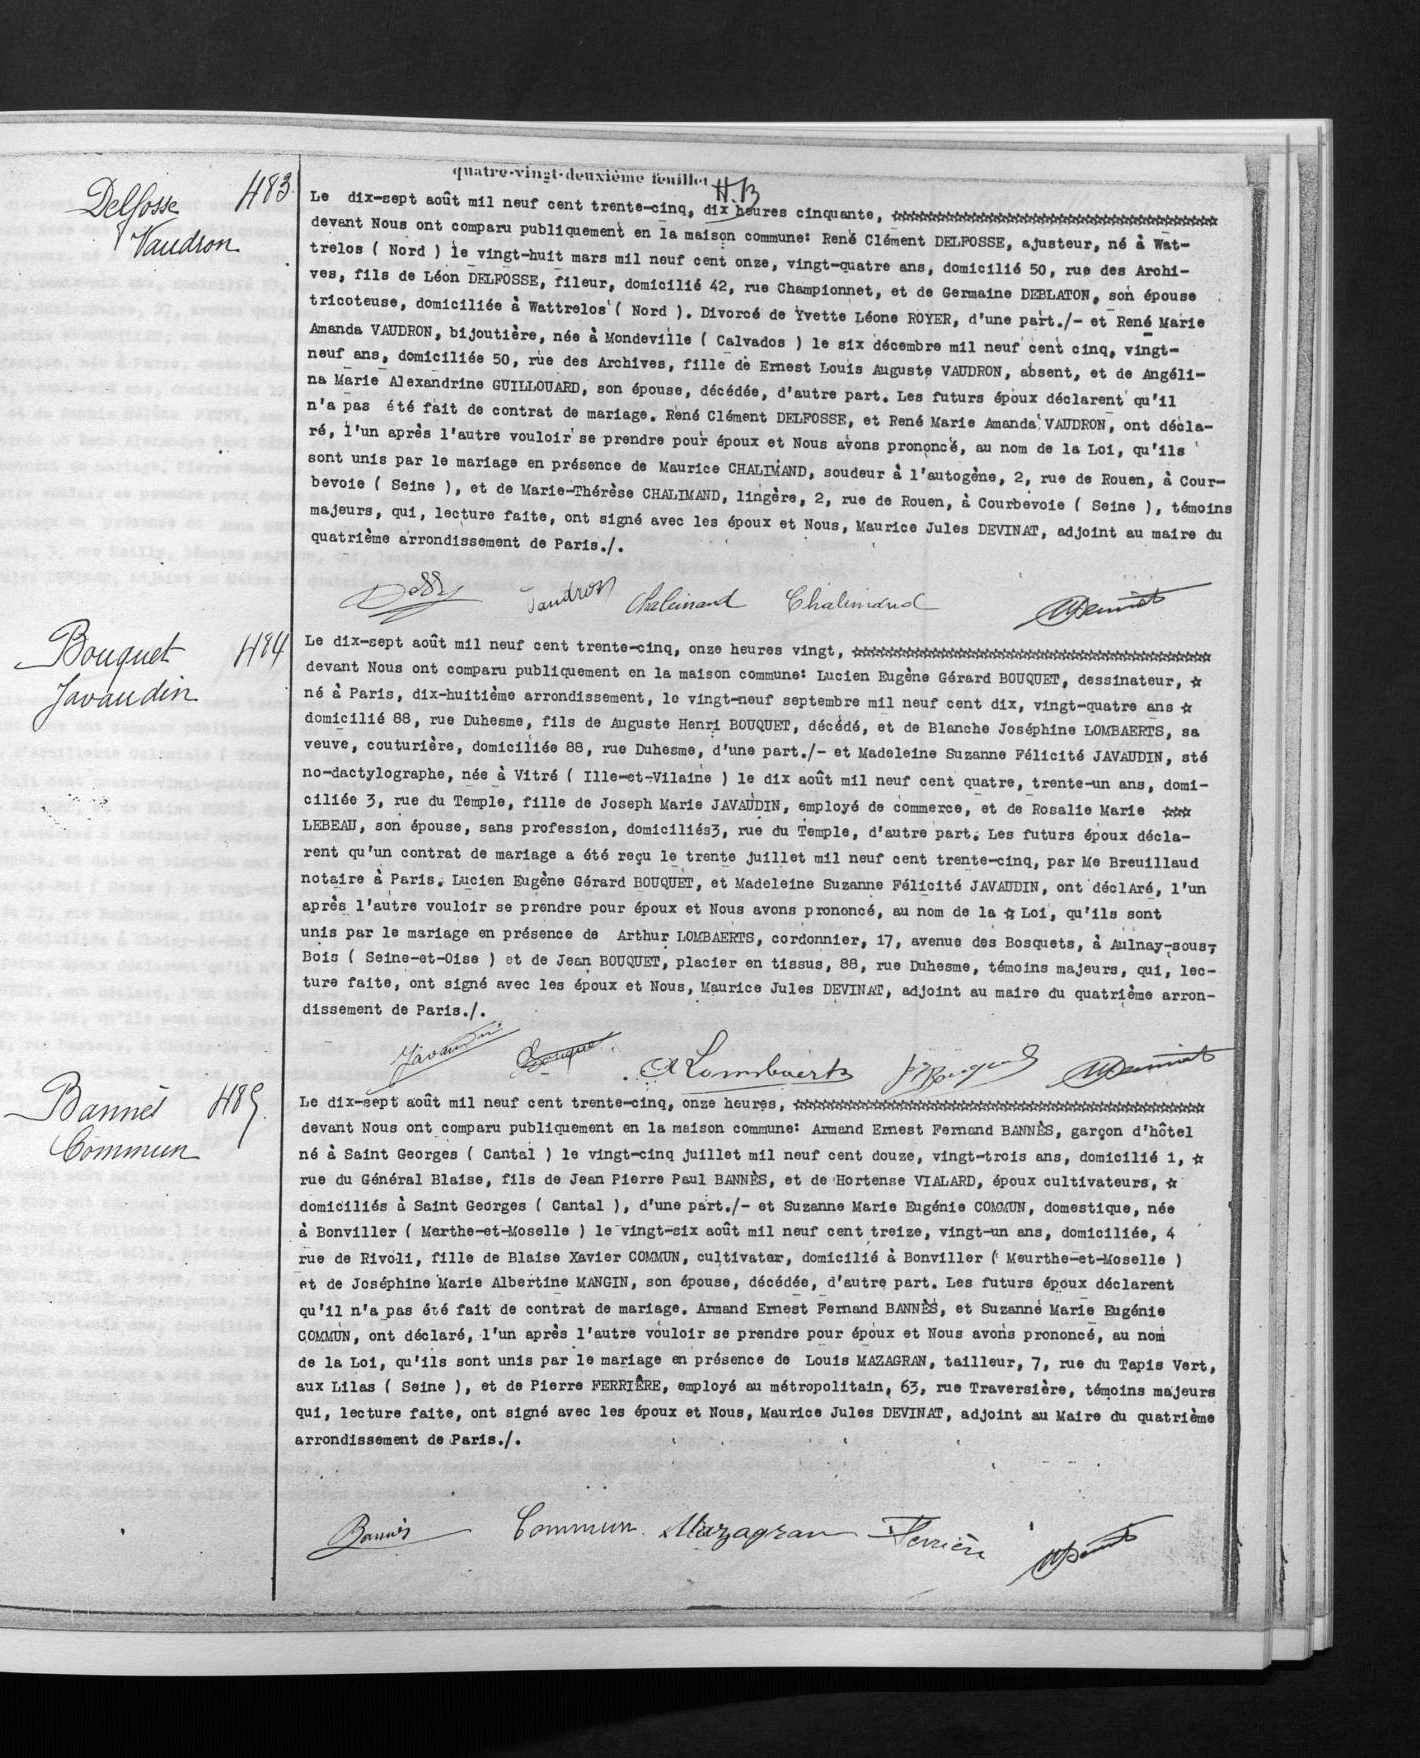Le dix-sept août mil neuf cent trente-cinq, dix heures cinquante, ****
devant Nous ont comparu publiquement en la maison commune: René Clément DELFOSSE, ajusteur, né à Wat-
trelos (Nord) le vingt-huit mars mil neuf cent onze, vingt-quatre ans, domicilié 50, rue des Archi-
ves, fils de Léon DELFOSSE, fileur, domicilié 42, rue Championnet, et de Germaine DEBLATON, son épouse
tricoteuse, domiciliée à Wattrelos (Nord). Divorcé de Yvette Léone ROYER, d'une part ./-et René Marie
Amanda VAUDRON, bijoutière, née à Mondeville (Calvados) le six décembre mil neuf cent cinq, vingt-
neuf ans, domiciliée 50, rue des Archives, fille de Ernest Louis Auguste VAUDRON, absent, et de Angéli-
na Marie Alexandring GUILLOUARD, son épouse, décédée, d'autre part. Les futurs époux déclarent qu'il
n'a pas été fait de contrat de mariage. René Clément DELFOSSE, et René Marie Amanda VAUDRON, ont décla-
ré, l'un après l'autre vouloir se prendre pour époux et Nous avons pronnoncé, au nom de la Loi, qu'ils
sont unis par le mariage en présence de Maurice CHALIMAND, soudeur à l'autogène, 2, rue de Rouen, à Cour-
bevoie (Seine), et de Marie-Thérèse CHALIMAND, lingère, 2, rue de Rouen, à Courbevoie (Seine), témoins
majeurs, qui, lecture faite, ont signé avec les époux et Nous, Maurice Jules DEVINAT, adjoint au maire du
quatrième arrondissement de Paris ./.
Le dix-sept août mil neuf cent trente-cinq, onze heures vingt, ****
devant Nous ont comparu publiquement en la maison commune: Lucien Eugène Gérard BOUQUET, dessinateur, *
né à Paris, dix-huitième arrondissement, le vingt-neuf septembre mil neuf cent dix, vingt-quatre ans *
domicilié 88, rue Duhesme, fils de Auguste Henri BOUQUET, décédé, et de Blanche Joséphine LOMBAERTS, sa
veuve, couturière, domiciliée 88, rue Duhesme, d'une part ./-et Madeleine Suzanne Félicité JAVAUDIN, sté
no-dactylographe, née à Vitré (Ille-et-Vilaine) le dix-août mil neuf cent quatre, trente-un ans, domi-
ciliée 3, rue du Temple, fille de Joseph Marie JAVAUDIN, employé de commerce, et de Rosalie Marie ***
LEBEAU, son épouse, sans profession, domiciliés 3, rue du Temple, d'autre part. Les futurs époux décla-
rent qu'un contrat de mariage a été reçu le trente juillet mil neuf cent trente-cinq, par Me Breuillaud
notaire à Pairs. Lucien Eugène Gérard BOUQUET, et Madeleine Suzanne Félicité JAVAUDIN, ont déclAré, l'un
après l'autre vouloir se prendre pour époux et Nous avons prononcé, an nom de la * Loi, qu'ils sont
unis par le mariage en présence de Arthur LOMBAERTS, cordonnier, 17, avenue des Bosquets, à Aulnay-sous -,
Bois (Seine-et-Oise) et de Jean BOUQUET, placier en tissus, 88, rue Duhesme, témoins majeurs, qui, lec-
ture faite, ont signé avec les époux et Nous, Maurice Jules DEVINAT, adjoint au maire du quatrième arron-
dissement de Pairs ./.
Le dix-sept août mil neuf cent trente-cinq, onze heures, ****
devant Nous ont comparu publiquement en la maison commune: Armand Ernest Fernand BANNES, garçon d'hôtel
né à Saint Georges (Cantal) le vingt-cinq juillet mil neuf cent douze, vingt-trois ans, domicilié 1, *
rue du Général Blaise, fils de Jean Pierre Paul BANNES, et de Hortense VIALARD, époux cultivateurs, *
domiciliés à Sant Georges (Cantal), d'une part ./-et Suzanne Marie Eugénie COMMUN, domestique, née
à Bonvillier (Marthe-et-Moselle) le vingt-six août mil neuf cent treize, vingt-un ans, domiciliée, 4
rue de Rivoli, fille de Blaise Xavier COMMUN, cultivatar, domicilié à Bonvillier (Meurthe-et-Moselle)
et de Joséphine Marie Albertine MANGIN, son épouse, décédée, d'autre part. Les futurs époux déclarent
qu'il n'a pas été fait de contrat de mariage. Armand Ernest Fernand BANNES, et Suzanne Marie Eugénie
COMMUN, ont déclaré, l'un après l'autre vouloir se prendre pour époux et Nous avons prononcé, au nom
de la Loi, qu'ils sont unis par le mariage en présence de Louis MAZAGRAN, tailleur, 7, rue du Tapis Vert,
aux Lilas (Seine), et de Pierre FERRIERE, employé au métropolitain, 63, rue Traversière, témoins majeurs
qui, lecture faite, ont signé avec les époux et Nous, Maurice Jules DEVINAT, adjoint au Maire du quatrième
arrondissement de Paris ./.
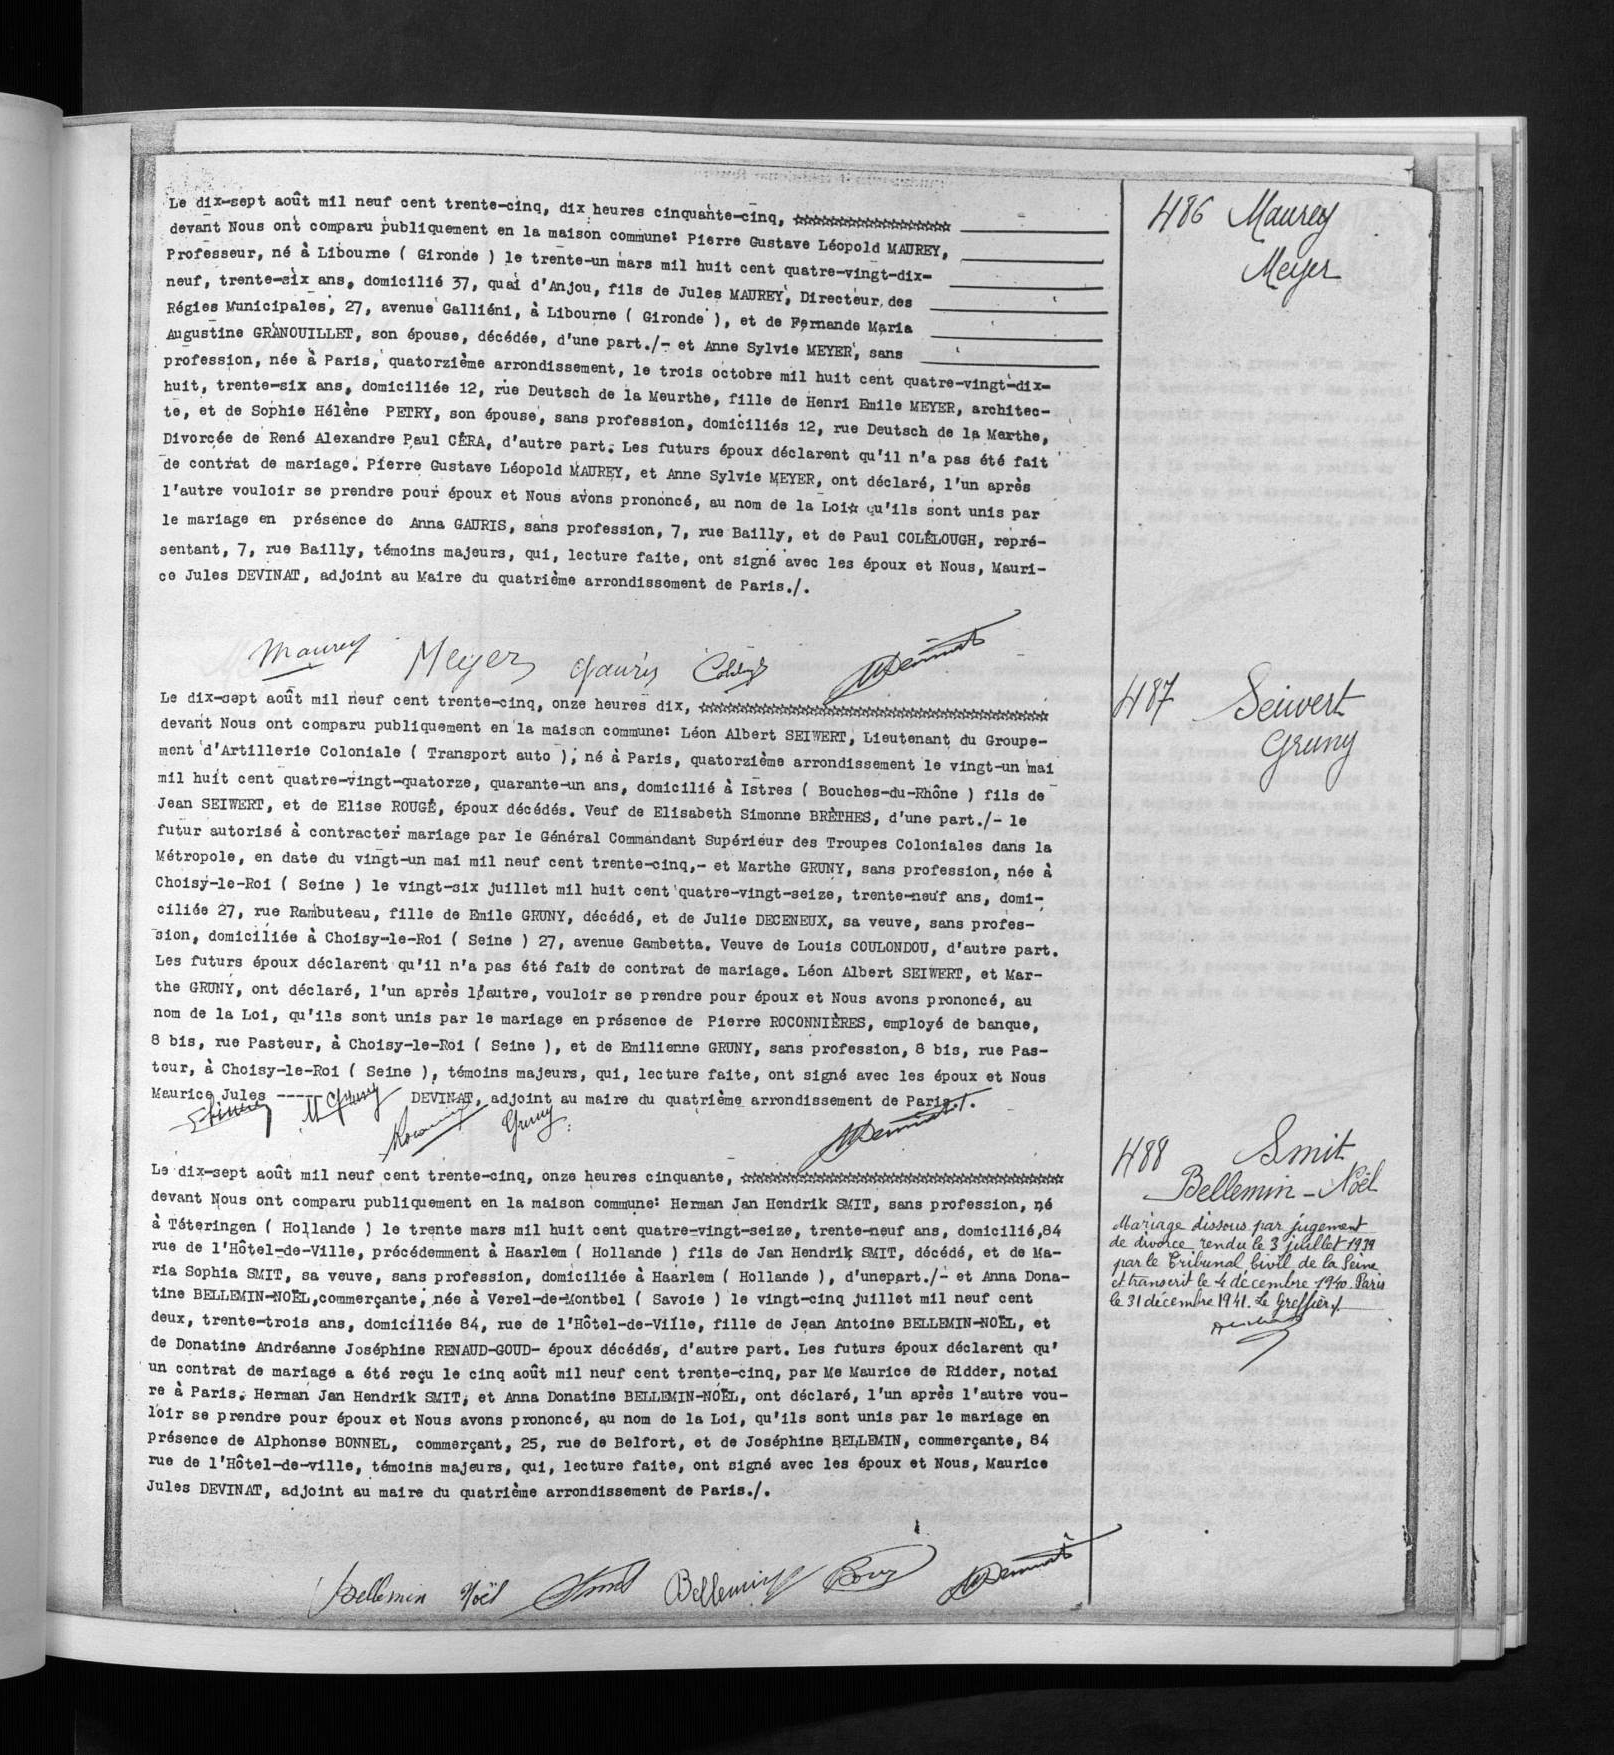Le dix-sept août mil neuf cent trente-cinq, dix heures cinquante-cinq, ****-
devant Nous ont comparu publiquement en la maison commune: Pierre Gustave Léopold MAUREY ,-
Professeur, né à Libourne (Gironde) le trente-un mars mil huit cent quatre-vingt-dix -
neuf, trente-six ans, domicilié 37, quai d'Anjou, fils de Jules MAUREY, Directeur des -
Régies Municipales, 27, avenue Galliéni, à Libourne (Gironde), et de Fernande Maria -
Augustine GRANOUILLET, son épouse, décédée, d'une part ./-et Anne Sylvie MEYER, sans -
profession, née à Paris, quatorzième arrondissement, le trois octobre mil huit cent quatre-vingt-dix-
huit, trente-six ans, domiciliée 12, rue Deutsch de la Meurthe, fille de Henri Emile MEYER, architec-
te, et de Sophie Hélène PETRY, son épouse, sans profession, domiciliés 12, rue Deutsch de la Marthe,
Divorcée de René Alexandre Paul CERA, d'autre part. Les futurs époux déclarent qu'il n'a pas été fait
de contrat de mariage. Pierre Gustave Léopold MAUREY, et Anne Sylvie MEYER, ont déclaré, l'un après
l'autre vouloir se prendre pour époux et Nous avons prononcé, au nom de la Loi * qu'ils sont unis par
le mariage en présence de Anna GAURIS, sans profession, 7, rue Bailly, et de Paul COLELOUGH, repré-
sentant, 7, rue Bailly, témoins majeurs, qui, lecture faite, ont signé avec les époux et Nous, Mauri-
ce Jules DEVINAT, adjoint au Maire du quatrième arrondissement de Paris ./.
Le dix-sept août mil neuf cent trente-cinq, onze heures dix, ****
devant Nous ont comparu publiquement en la maison commune: Léon Albert SEIWERT, Lieutenant du Groupe-
ment d'Artillerie Coloniale (Transport auto), né à Paris, quatorzième arrondissement le vingt-un mai
mil huit cent quatre-vingt-quatorze, quarante-un ans, domicilié à Istres (Bouches-du-Rhône) fils de
Jean SEIWERT, et de Elise ROUGE, époux décédés. Veuf de Elisabeth Simonne BRETHES, d'une part ./-le
futur autorisé à contracter mariage par le Général Commandant Supérieur des Troupes Coloniales dans la
Métropole, en date du vingt-un mai mil neuf cent trente-cinq ,-et Marthe GRUNY, sans profession, née à
Choisy-le-Roi (Seine) le vingt-six juillet mil huit cent quatre-vingt-seize, trente-neuf ans, domi-
ciliée 27, rue Rambuteau, fille de Emile GRUNY, décédé, et de Julie DECENEUX, sa veuve, sans profes-
sion, domiciliée à Choisy-le-Roi (Seine) 27, avenue Gambette. Veuve de Louis COULANDOU, d'autre part.
Les futurs époux déclarent qu'il n'a pas été fait de contrat de mariage. Léon Albert SEIWERT, et Mar-
the GRUNY, ont déclaré, l'un après l'autre, vouloir se prendre pour époux et nous avons prononcé, au
nom de la Loi, qu'ils sont unis par le mariage en présence de Pierre ROCONNIERES, employé de banque,
8 bis, rue Pasteur, à Choisy-le-Roi (Seine), et de Emilienne GRUNY, sans profession, 8 bis, rue Pas-
tour, à Choisy-le-Roi (Seine), témoins majeurs, qui, lecture faite, ont signé avec les époux et Nous
Maurice Jules -DEVINAT, adjoint au maire du quatrième arrondissement de Paris ./.
Le dix-sept août mil neuf cent trente-cinq, onze heures cinquante, ****
devant Nous ont comparu publiquement en la maison commune: Herman Jan Hendrik SMIT, sans profession, né
à Teteringen (Hollande) le trente mars mil huit cent quatre-vingt-seize, trente-neuf ans, domicilié, 84
rue de l'Hôtel-de-Ville, précédemment à Haarlem (Hollande) fils de Jan Hendrik SMIT, décédé, et de Ma-
ria Sophia SMIT, sa veuve, sans profession, domiciliée à Haarlem (Hollande), d'une part ./-et Anna Dona-
tine BELLEMIN-NOEL, commerçante, née à Verel-de-Montbel (Savoie) le vingt-cinq juillet mil neuf cent
deux, trente-trois ans, domicilié 84, rue de l'Hôtel-de-Ville, fille de Jean Antoine BELLEMIN-NOEL, et
de Donastine Andréanne Joséphine RENAUD-GOUD-époux décédés, d'autre part. Les futurs époux déclarent qu'
un contrat de mariage a été reçu le cinq août mil neuf cent trente-cinq, par Me Maurice de Ridder, notai
re à Paris. Jerman Jan Hendrik SMIT, et Anna Donatine BELLEMIN-NOEL, ont déclaré, l'un après l'autre vou-
loir se prendre pour époux et Nous avons pronnoncé, au nom de la Loi, qu'ils sont unis par le mariage en
présence de Alphonse BONNEL, commerçcant, 25, rue de Belfort, et de Joséphine BELLEMIN, commerçante, 84
rue de l'Hôtel-de-ville, témoins majeurs, qui, lecture faite, ont signé avec les époux et Nous, Maurice
Jules DEVINAT, adjoint au maire du quatrième arrondissement de Paris ./.